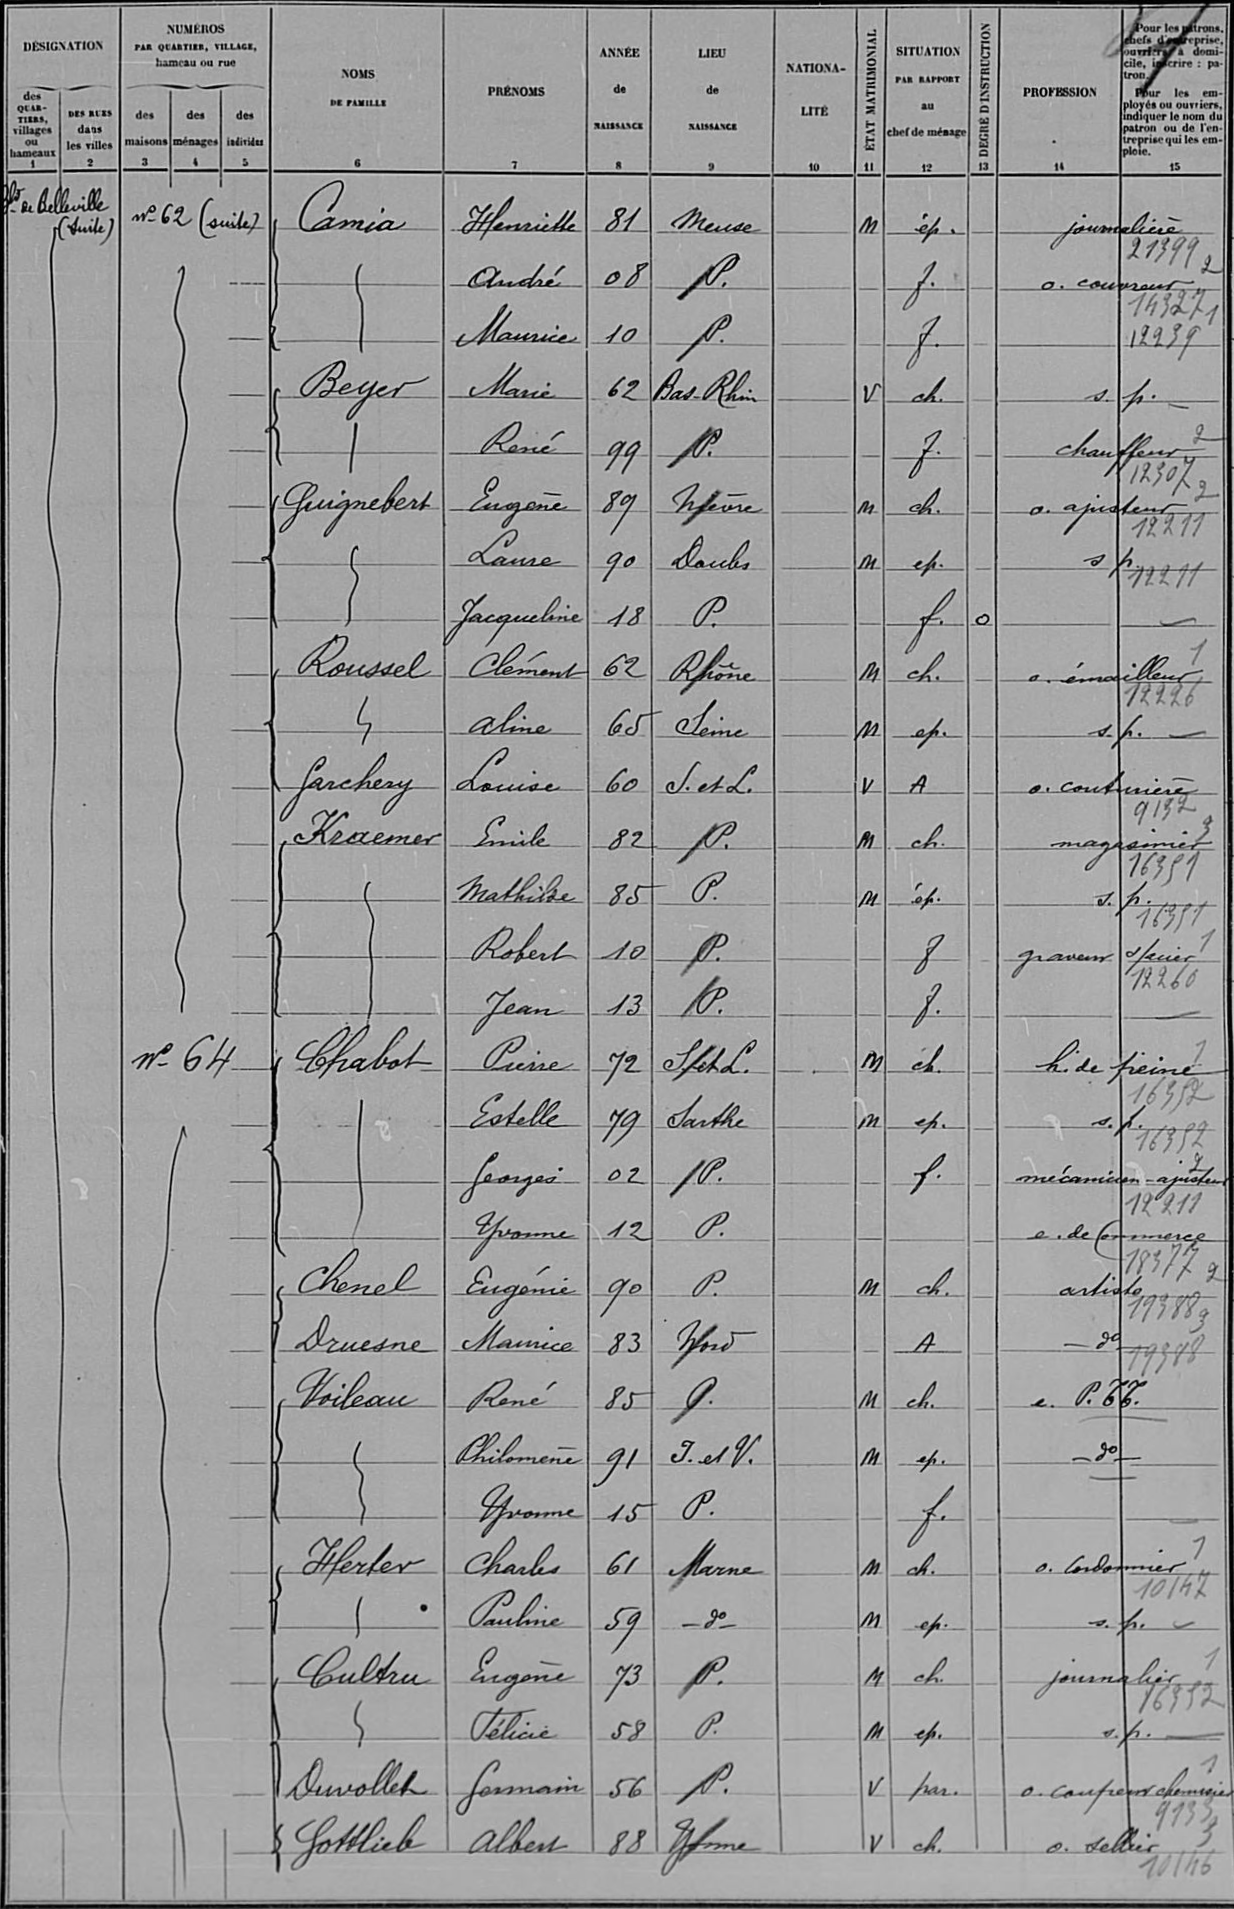Camia/Henriette/81/Meuse/¤/M/ép /¤/journalière/¤
¤/André/08/P /¤/¤/f /¤/o couvreur/¤
¤/Maurice/10/P /¤/¤/f /¤/¤/12239
Beyer/Marie/62/Bas-Rhin/¤/V/ch /¤/s p /¤
¤/René/99/P /¤/¤/f /¤/chauffeur/¤
Guignebert/Eugène/89/Nièvre/¤/M/ch /¤/o ajusteur/¤
¤/Laure/90/Doubs/¤/M/ep /¤/sp/¤
¤/Jacqueline/18/P /¤/¤/f /0/¤/¤
Roussel/Clément/62/Rhône/¤/M/ch /¤/o émailleur/¤
¤/Aline/65/Seine/¤/M/ep /¤/s p /¤
Garchery/Louise/60/S et L /¤/V/A/¤/o couturière/¤
Kraemer/Emile/82/P /¤/M/ch /¤/magasinier/¤
¤/MAthilde/85/P /¤/M/ép /¤/s p /¤
¤/Robert/10/P /¤/¤/f/¤/graveur s acier/¤
¤/Jean/13/P /¤/¤/f /¤/¤/¤
Chabot/Pierre/72/S et L /¤/M/ch /¤/h de peine/¤
¤/Estelle/79/Sarthe/¤/M/ep /¤/s p /¤
¤/Georges/02/P /¤/¤/f /¤/mécanicienne-ajusteur/¤
¤/Yvonne/12/P /¤/¤/¤/¤/e de commerce/¤
Chenel/Eugénie/90/P /¤/M/ch /¤/artiste/¤
Druesne/Maurice/83/Nord/¤/¤/A/¤/-d°/¤
Voileau/René/85/P /¤/M/ch /¤/e P T T /¤
¤/Philomène/91/I et V /¤/M/ep /¤/d°/¤
¤/Yvonne/15/P /¤/¤/f /¤/¤/¤
Herter/Charles/61/Marne/¤/ch /¤/o cordonnier/¤
¤/Pauline/59/d°/¤/M/ep /¤/s p /¤
Cultru/Eugène/73/P /¤/M/ch /¤/journalier/¤
¤/Félicie/58/P /¤/M/ep /s p /¤
Duvollet/germain/56/P /¤/V/par /¤/o coupeur chemies/¤
Gottlieb/Albert/88/Yonne/¤/V/ch /¤/o sellier/¤